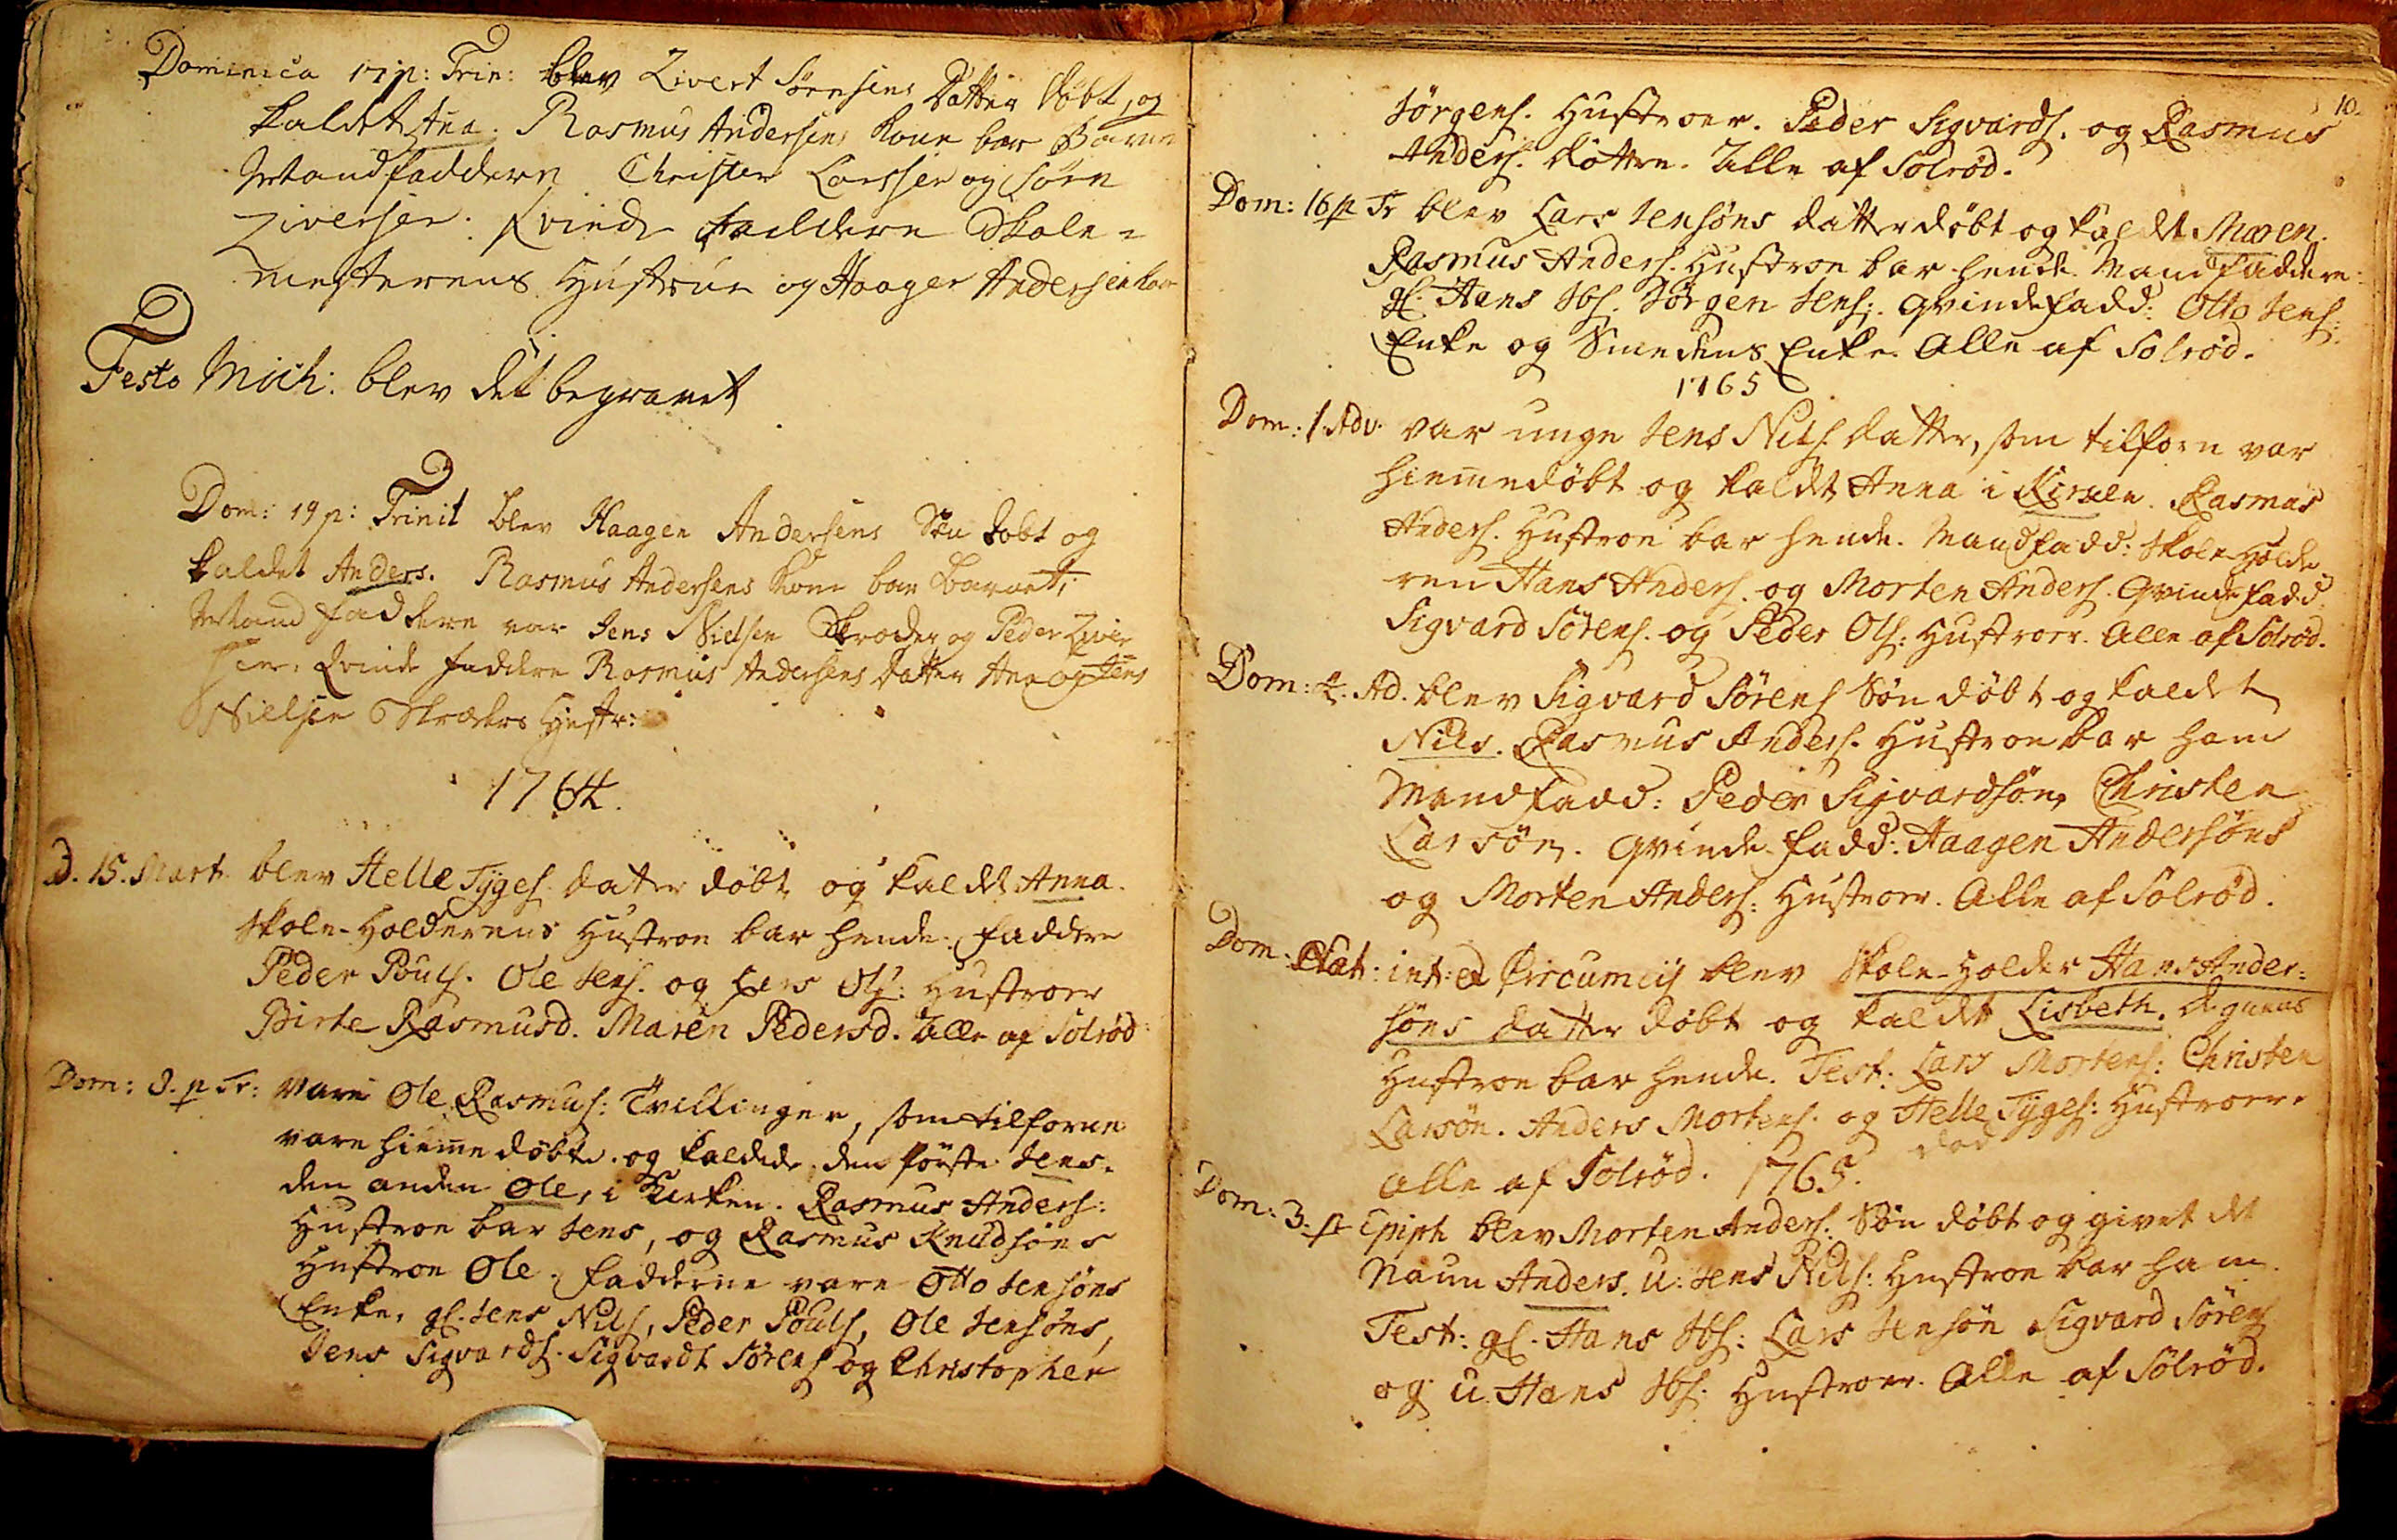Dominica 17 p: Trin: blev Zivert Sørnsens Datter døbt, og
kaldet Ana. Rasmus Andersens Kone bar Barnet
Mandfaddere. Christen Larssen og Sørn
Ziversen. Qvinde faddere Skole¬
mesterens Hustrue og Haagen Andersens Kone
Festo Mich: blev det begravet.
Dom: 19 p: Trinit blev Haagen Andersens Søn døbt og
kaldet Anders. Rasmus Andersens Kone bar Barnet;
Mand faddere var Jens Nielsen Skræder og Peder Ziver¬
sen. Qvinde faddere Rasmus Andersens Datter Ana og Jens
Nielsen Skræders Hustr:
1764
d. 15. Mart: blev Helle Tyges. Datter døbt og kaldt Anna.
Skole-Holderens Hustroe bar hende. faddere
Peder Pouls. Ole Jens. og Lars Ols: Hustroer
Birte Rasmusd. Maren Pedersd. Alle af Solrød
Dom: 3. p Tr: vare Ole Rasmus: Tvillinger, som tilfor##en
vare hiemedøbte og kaldede, den første Jens.
den anden Ole, i Kirken. Rasmus Anders:
Hustroe bar Jens, og Rasmus Knudsøns
Hustroe Ole: fadderne vare Otto Jensøns
Enke, gl. Jens Nils, Peder Pouls, Ole Jensøns,
Jens Sigvards. Sigvardt Sørens og Christopher
10.
Jørgens. Hustroer. Peder Sigvards. og Rasmus
Anders. Døttre. Alle af Solrød.
Dom: 16 p Tr blev Lars Jensøns Datter døbt og kaldt Maren.
Rasmus Anders. Hustroe bar hende. Mandfaddere
gl. Hans Ibs. Jørgen Jens:. Qvindefadd:. Otto Jens:
Enke og Smedens Enke. Alle af Solrød.
1765.
Dom: 1 Adv. var unge Jens Nils Datter, som tilforn var
hiemedøbt og kaldt Anna i Kirken. Rasmus
Anders. Hustroe bar hende. Mandfadd: Skole-Holde
ren Hans Anders. og Morten Anders. Qvindefadd:
Sigvard Sørens. og Peder Ols: Hustroer. Alle af Solrød
Dom: 4. Ad. blev Sigvard Sørens Søn døbt og kaldt
Nils. Rasmus Anders. Hustroe bar ham
Mandfadd: Peder Sigvardsøn, Christen
Larsøn. Qvinde-fadd: Haagen Andersøns
og Morten Anders: Hustroer. Alle af Solrød.
Dom. Nat: Int: ex Circumcis blev Skole-Holder Hans Ander¬
søns Datter døbt og kaldt Lisbeth. Degnens
Hustroe bar hende. Test. Lars Mortens: Christen
Larsøn. Anders Mortens. og Helle Tyges. Hustroer.
Alle af Solrød.
død.
1765.
Dom: 3. p Epiph blev Morten Anders. Søn døbt og givet det
naun Anders. u: Jens Nils: Hustroe bar ham.
Test: gl. Hans Ibs: Lars Jensøn Sigvard Sørens.
og u. Hans Ibs. Hustroer. Alle af Solrød
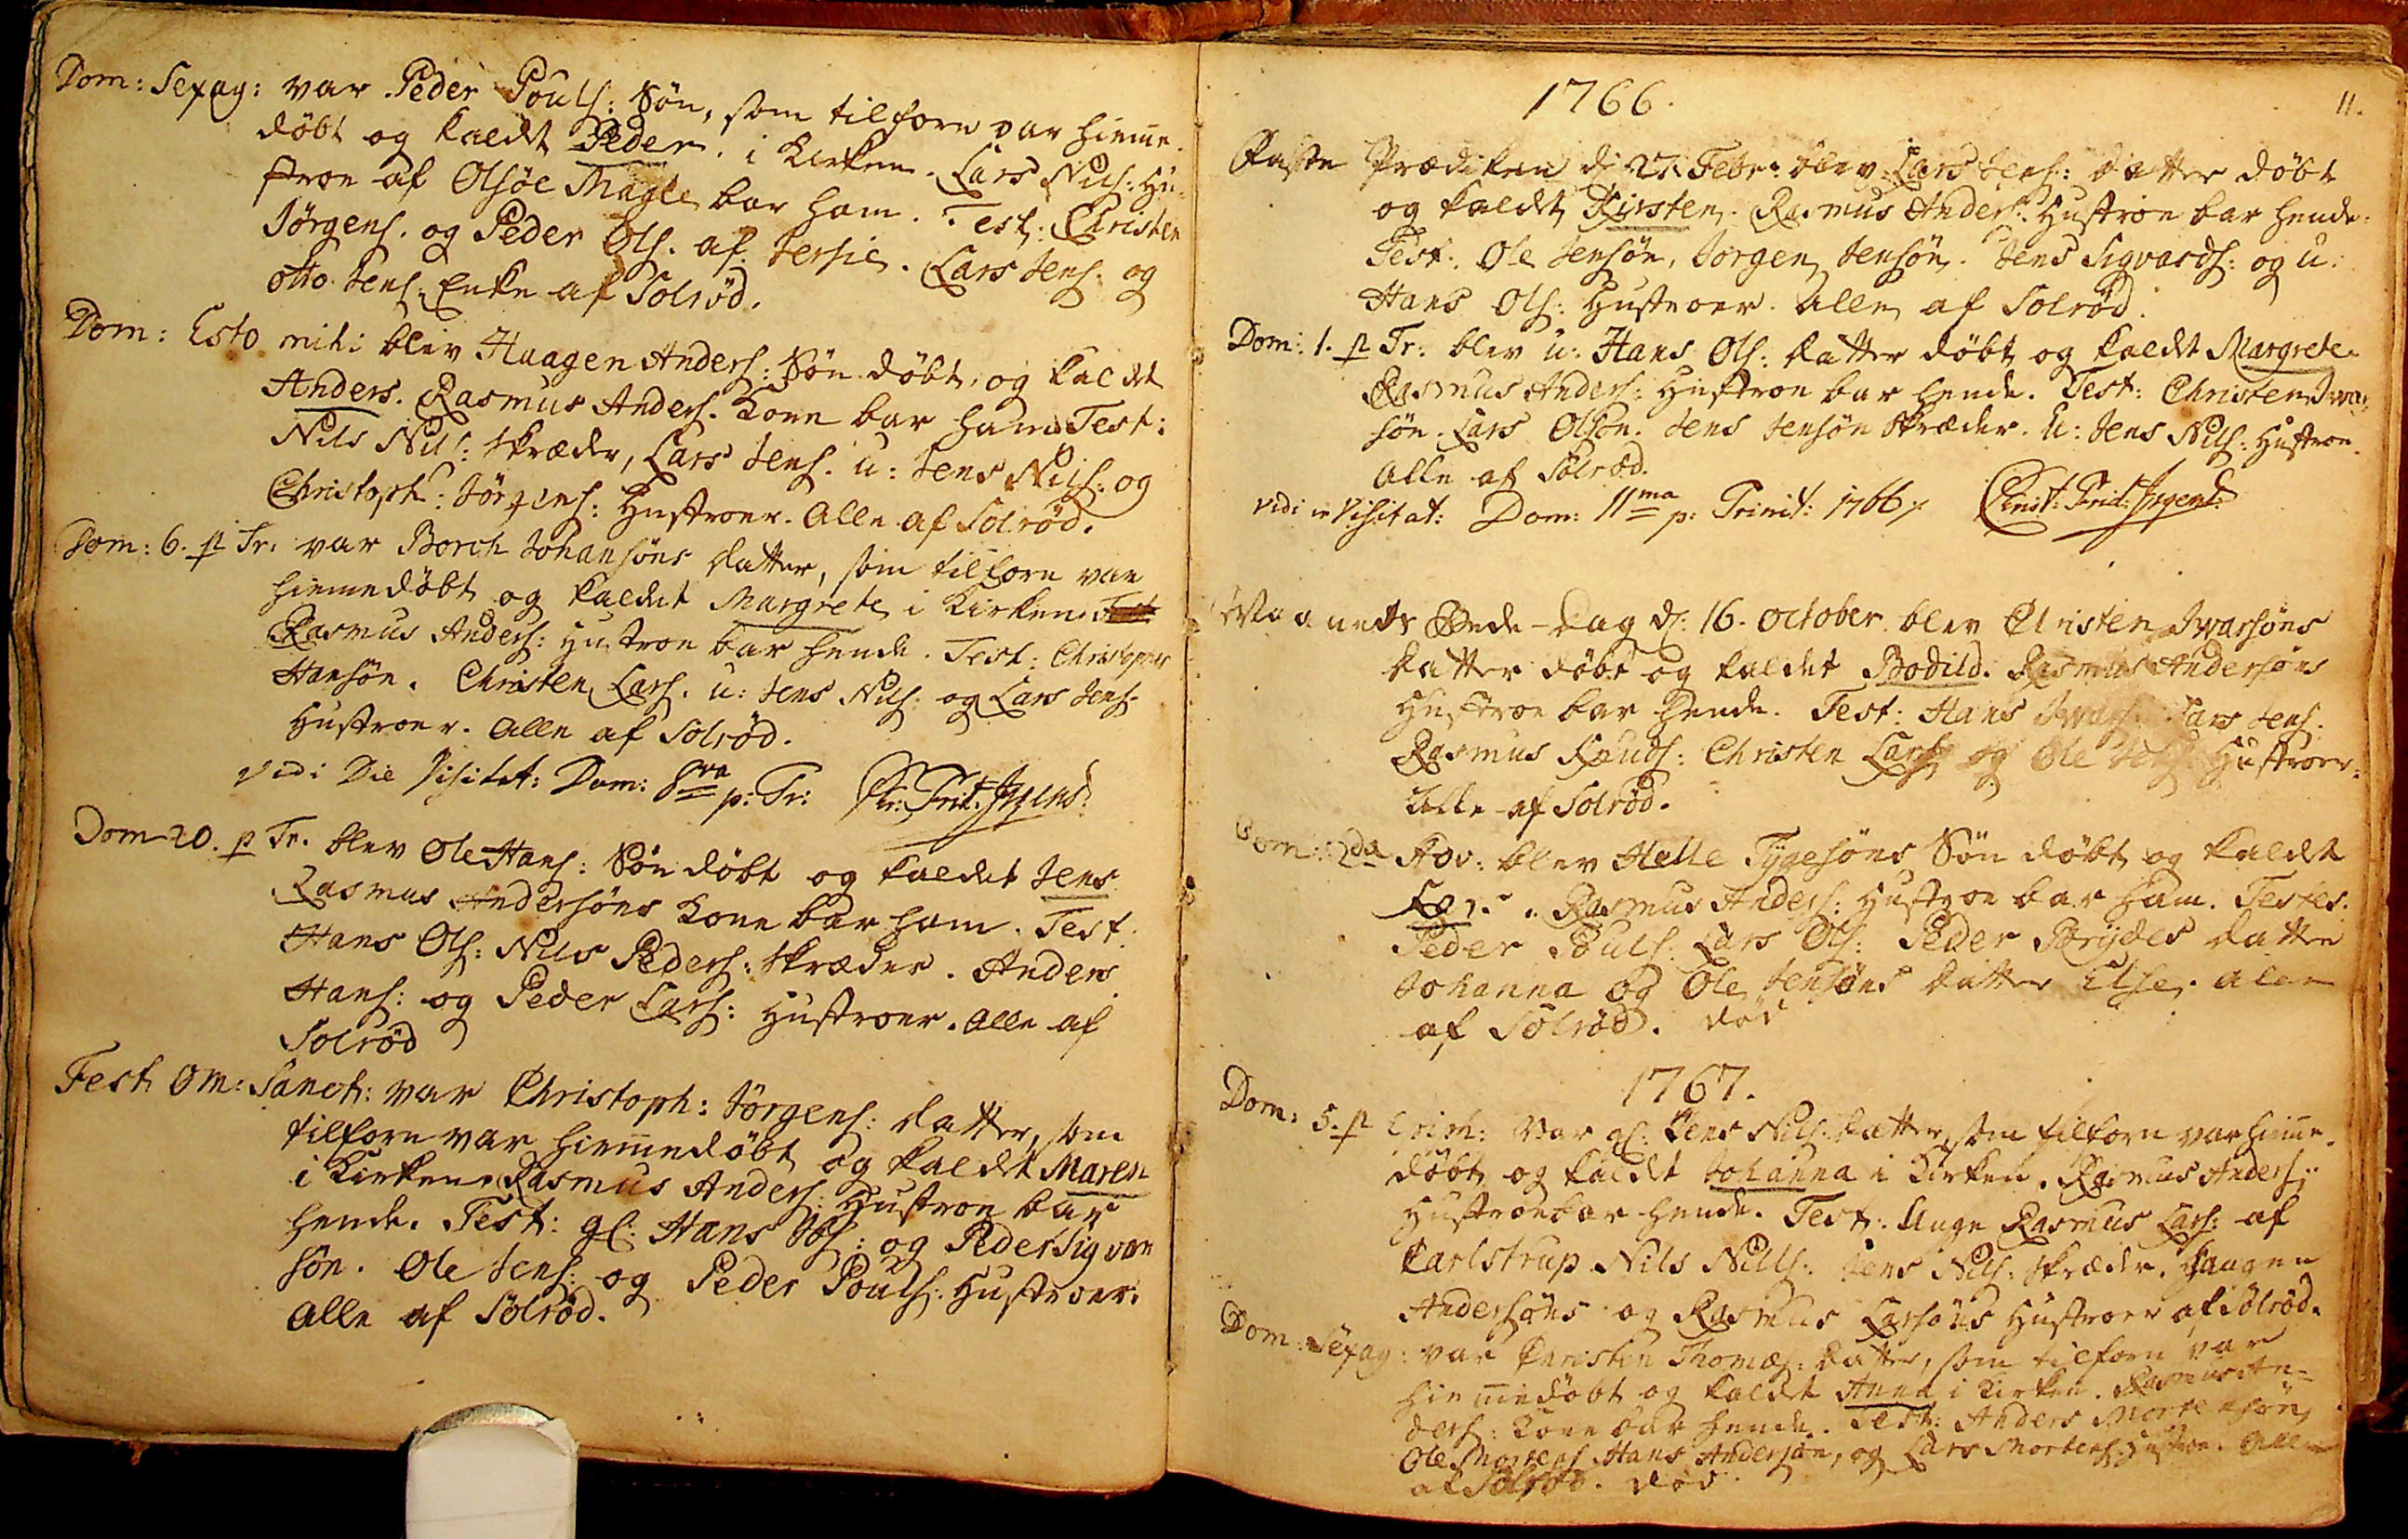Dom: Sexag: var Peder Pouls: Søn, som tilforn var hieme
døbt og kaldet Peder, i Kirken. Lars Nils: Hu¬
stroe af Ølsøe Magle bar ham. Test: Christen
Jørgens. og Peder Ols. af Jersie. Lars Jens. og
Otto Jens. Enke af Solrød.
Dom: Esto mihi blev Haagen Anders: Søn døbt, og kaldet
Anders. Rasmus Anders. Kone bar ham Test:
Niels Niels: Skræder, Lars Jens. U: Jens Nils: og
Christoph: Jørgens: Hustroer. Alle af Solrød.
Dom: 6. p Tr: var Borch Johansøns Datter, som tilforn var
hiemedøbt og kaldet Margrete i Kirken Test
Rasnus Anders: Hustroe bar hende. Test: Christopher
Hansøn. Christen Lars. u: Jens Nils: og Lars Jens.
Hustroer. Alle af Solrød.
Vidi Die Visitat: Dom: 8va p:Tr: Chr:Frid:Jrgens:
Dom 20. p Tr. blev Ole Hans: Søn døbt og kaldet Jens
Rasmus Andersøns Kone bar ham. Test.
Hans Ols: Nils Peders: Skræder. Anders
Hans: og Peder Lars: Hustroer. Alle af
Solrød
Fest Om: Sanct: var Christoph: Jørgens: Datter, som
tilforn var hiemedøbt og kaldet Maren
i Kirken. Rasmus Anders: Hustroe bar
hende. Test: gl: Hans Ibs: og Peder Sigvar
søn. Ole Jens: og Peder Pouls: Hustroer.
Alle af Solrød.
11.
1766
Faste Prædiken d 27. Febr: blev Lars Jens: Datter døbt
og kaldet Kirsten. Rasmus Anders: Hustroe bar hende:
Test: Ole Jensøn, Jørgen Jensøn. Jens Sigvards: og U:
Hans Ols: Hustroer. Alle af Solrød.
Dom: 1. p Tr: blev U: Hans Ols: Datter døbt og kaldet Margrete.
Rasmus Anders: Hustroe bar hende. Test: Christen Iwar
søn. Lars Olsøn. Jens Jensøn Skræder. U: Jens Nils: Hustroe
Alle af Solrød.
Vidi in Visitat: Dom: 11ma p: Trinit: 1766./. Christ: Frid: Jrgens:
Maaneds Bede-Dag d: 16. October blev Christen Iwarsøns
Datter døbt og kaldet Bodild. Rasmus Andersøns
Hustroe bar hende. Test: Hans Iwars: Lars Jens:
Rasmus Knuds: Christen Lars: og Ole Jens. Hustroer.
Alle af Solrød.
Dom: 2da Adv: blev Helle Tygesøns Søn døbt og kaldet
Lars. Rasmus Anders: Hustroe bar ham. Testes.
Peder Pouls: Lars Ols: Peder Brydes Datter
Johanna og Ole Jensøns Datter Else. Alle
af Solrød. død
1767.
Dom: 5. p Epiph: var gl: Jens Nils. Datter, som tilforn var hieme¬
døbt og kaldet Johanna i Kirken. Rasmus Anders.
Hustroe bar hende. Test: Unge Rasmus Lars: af
Carlstrup Nils Nils: Jens Nils: Skræder. Haagen
Andersøns og Rasmus Larsøns Hustroer af Solrød.
Dom: Sexag: var Christen Thomæs: Datter, som tilforn var
hiemedøbt og kaldet Anna i Kirken. Rasmus An¬
ders: Kone bar hende. Test: Anders Mortensøns
Ole Mortens. Hans Andersøn, og Lars Mortens: Hustroe. Alle
af Solrød. død